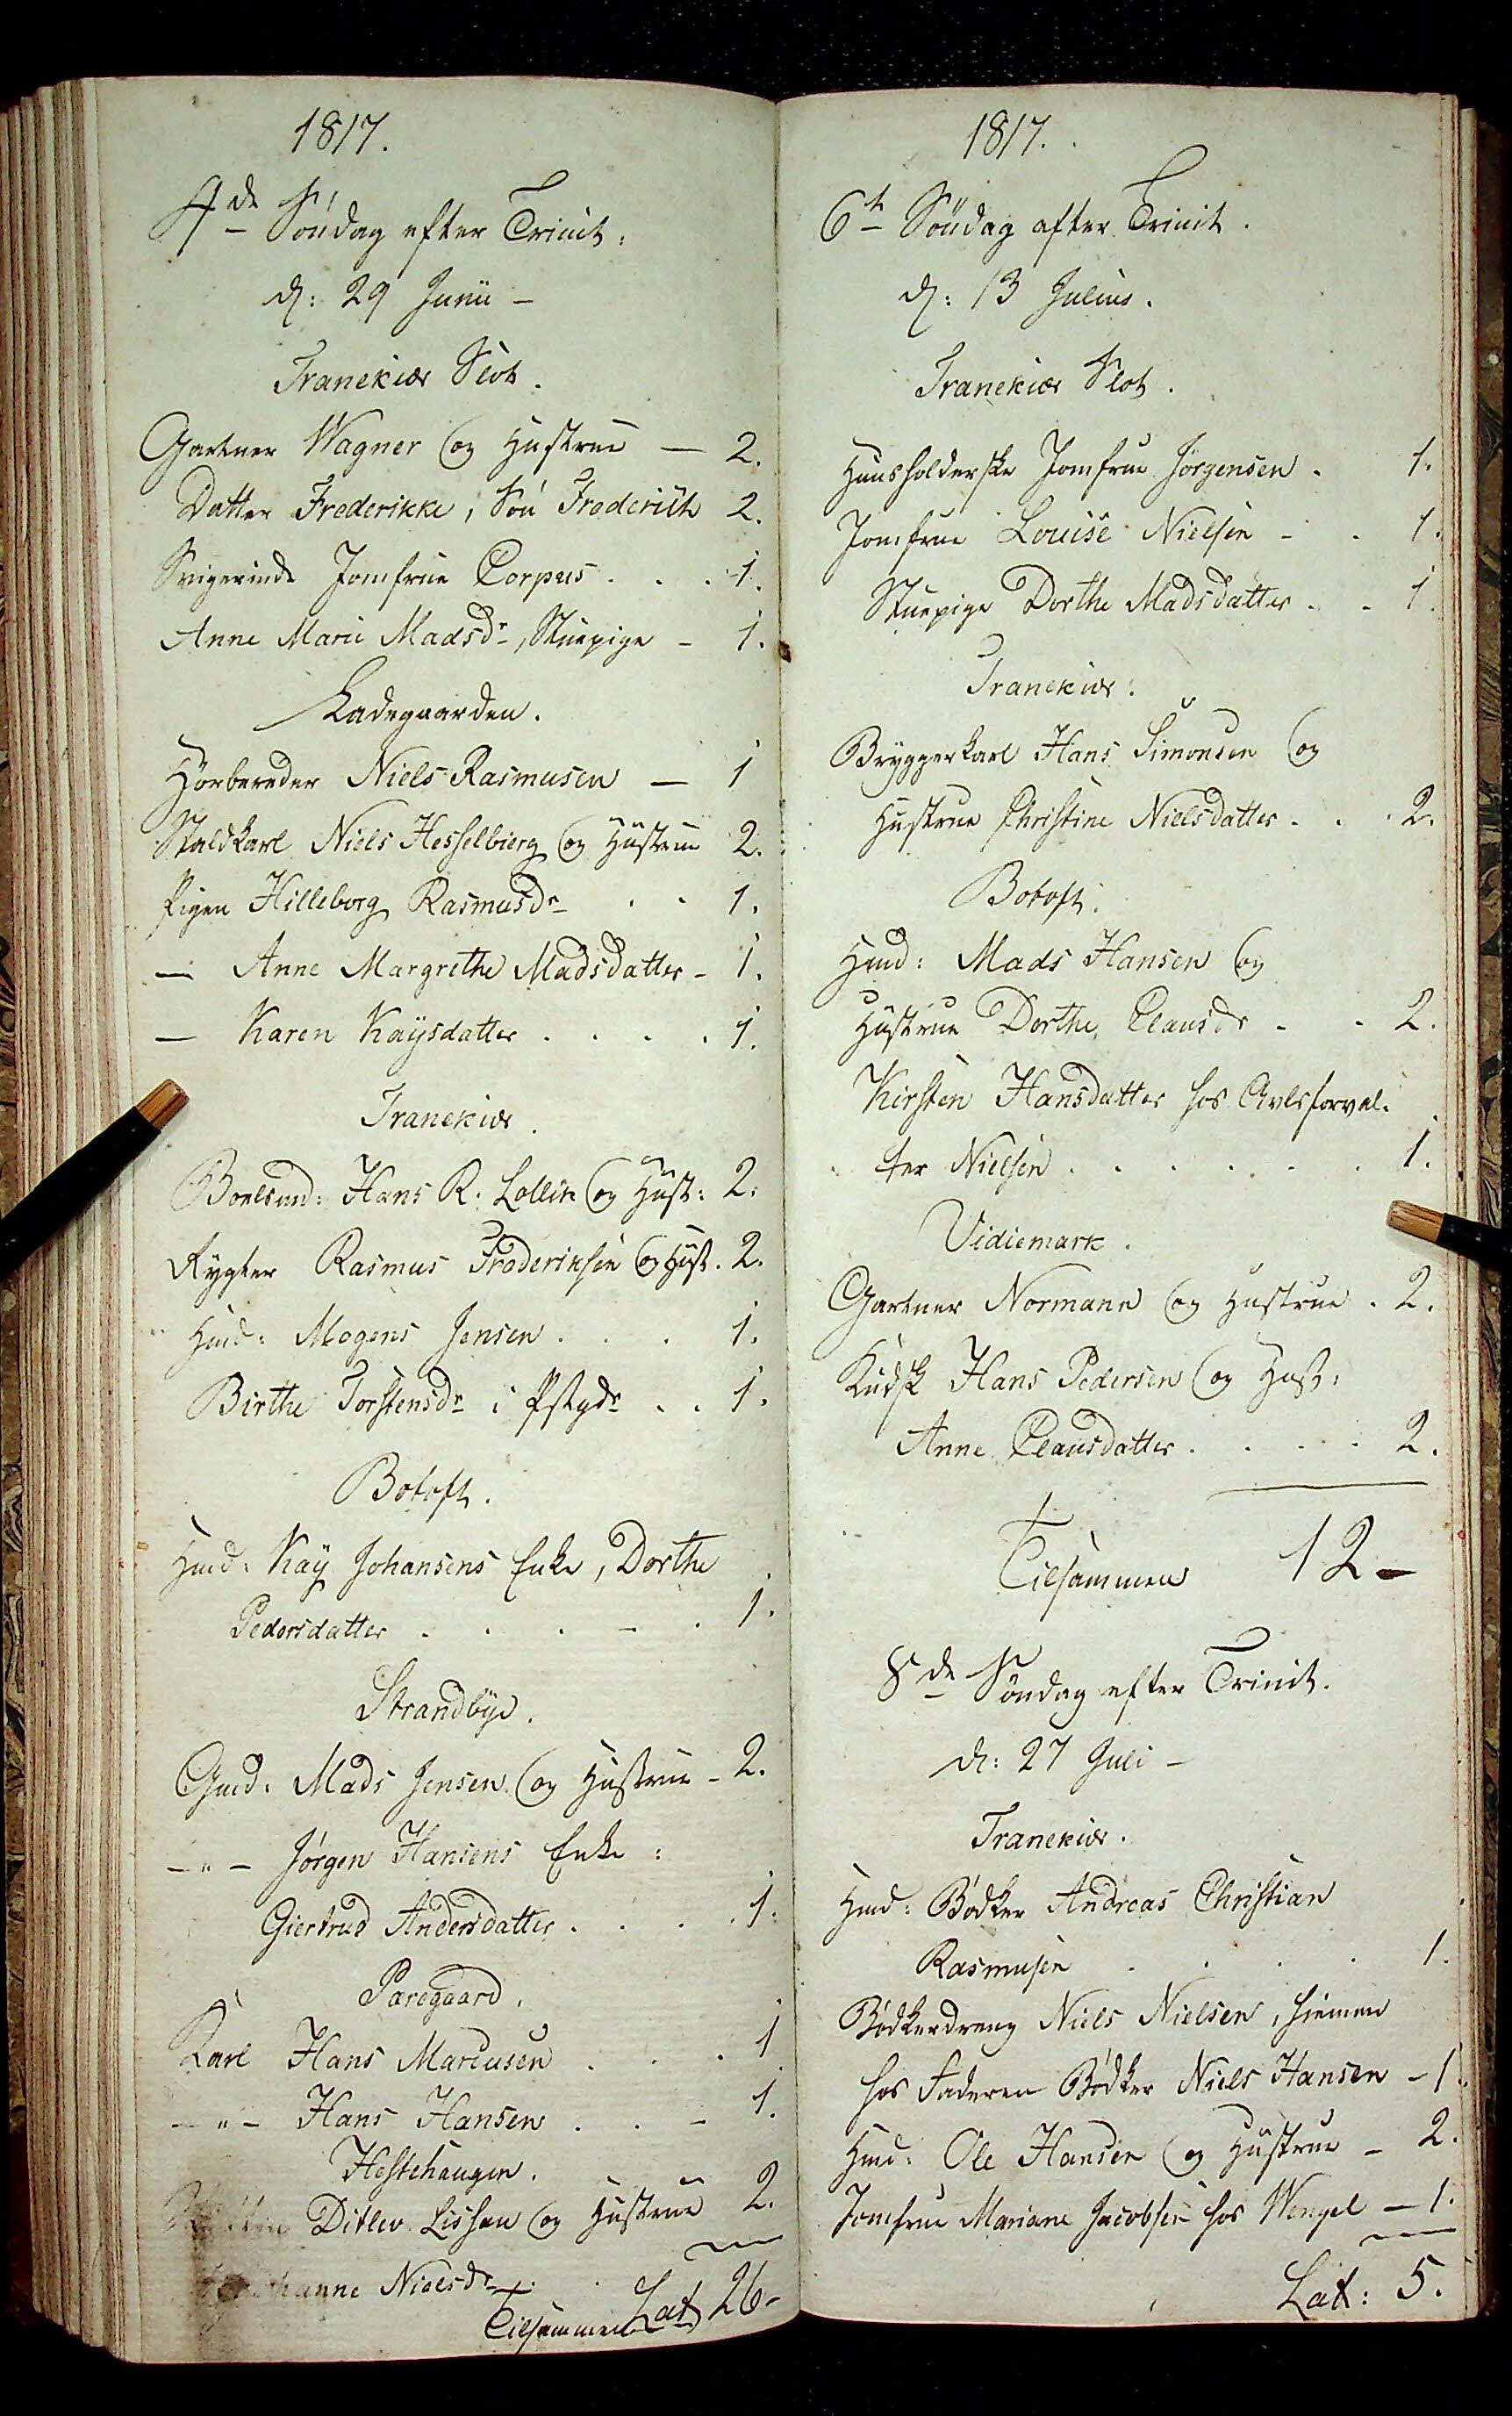1817.
4de Søndag efter Trinit:
d: 29 Junii
Tranekiær Slot.
Gartner Wagner og Hustrue - 2.
Datter Frederikke, Søn Friderich 2.
Svigerinde Jomfrue Corpus . . . 1.
Anne Marie Madsdr Stuepige - 1.
Ladegaarden.
Hørbereder Niels Rasmusen - 1
Staldkarl Niels Hesselbierg og Hustrue 2.
Pigen Hilleborg Rasmusdr . . 1.
- Anne Margrethe Madsdatter - 1.
- Karen Kaysdatter . . . . 1.
Tranekiær.
Boelsmd: Hans R. Lollik og Hust: 2.
Rygter Rasmus Frederiksen og Hust . 2.
Hmd: Mogens Jensen . . . 1
Birthe Torstensdr i Pstgdr . . 1.
Botoft.
Hmd: Kay Johansens Enke, Dorthe
Pedersdatter . . . - . 1
Strandbye.
Gmd: Mads Jensen og Hustrue - 2.
-"- Jørgen Hansens Enke:
Giertrud Andersdatter . . . . 1
Pæregaard.
Karl Hans Marcusen . . . 1
-"- Hans Hansen . . - 1.
Hestehaugen.
Skytten Ditlev Lissau og Hustrue 2.
## Johanne Nielsdr 
Tiksammen Lat 26-
1817.
6te Søndag efter Trinit.
d: 13 Juli##.
Tranekiær Slot.
Huusholderske Jomfrue Jørgensen . 1.
Jomfrue Louise Nielsen . . 1.
Stuepige Dorthe Madsdatter . . 1.
Tranekiær.
Bryggerkarl Hans Simonsen og
Hustrue Christine Nielsdatter . . 2.
Botoft.
Hmd: Mads Hansen og
Hustrue Dorthe Clausdr . . 2.
Kirsten Hansdatter hos Avlsforval¬
ter Nielsen . . . . . . . 1.
Vidiemark.
Gartner Normann og Hustrue . 2.
Kudsk Hans Pedersen og Hust:
Anne Clausdatter . . . . 2.
Tilisammen 12-
8de Søndag efter Trinit.
d: 27 Juli
Tranekiær.
Hmd: Bødker Andreas Christian
Rasmusen . . . . 1.
Bødkerdreng Niels Nielsen, hiemme
hos Faderen Bødker Niels Hansen -1
Hmd: Ole Hansen og Hustrue - 2
Jomfrue Mariane Jacobsen hos Wengel - 1
Lat: 5.
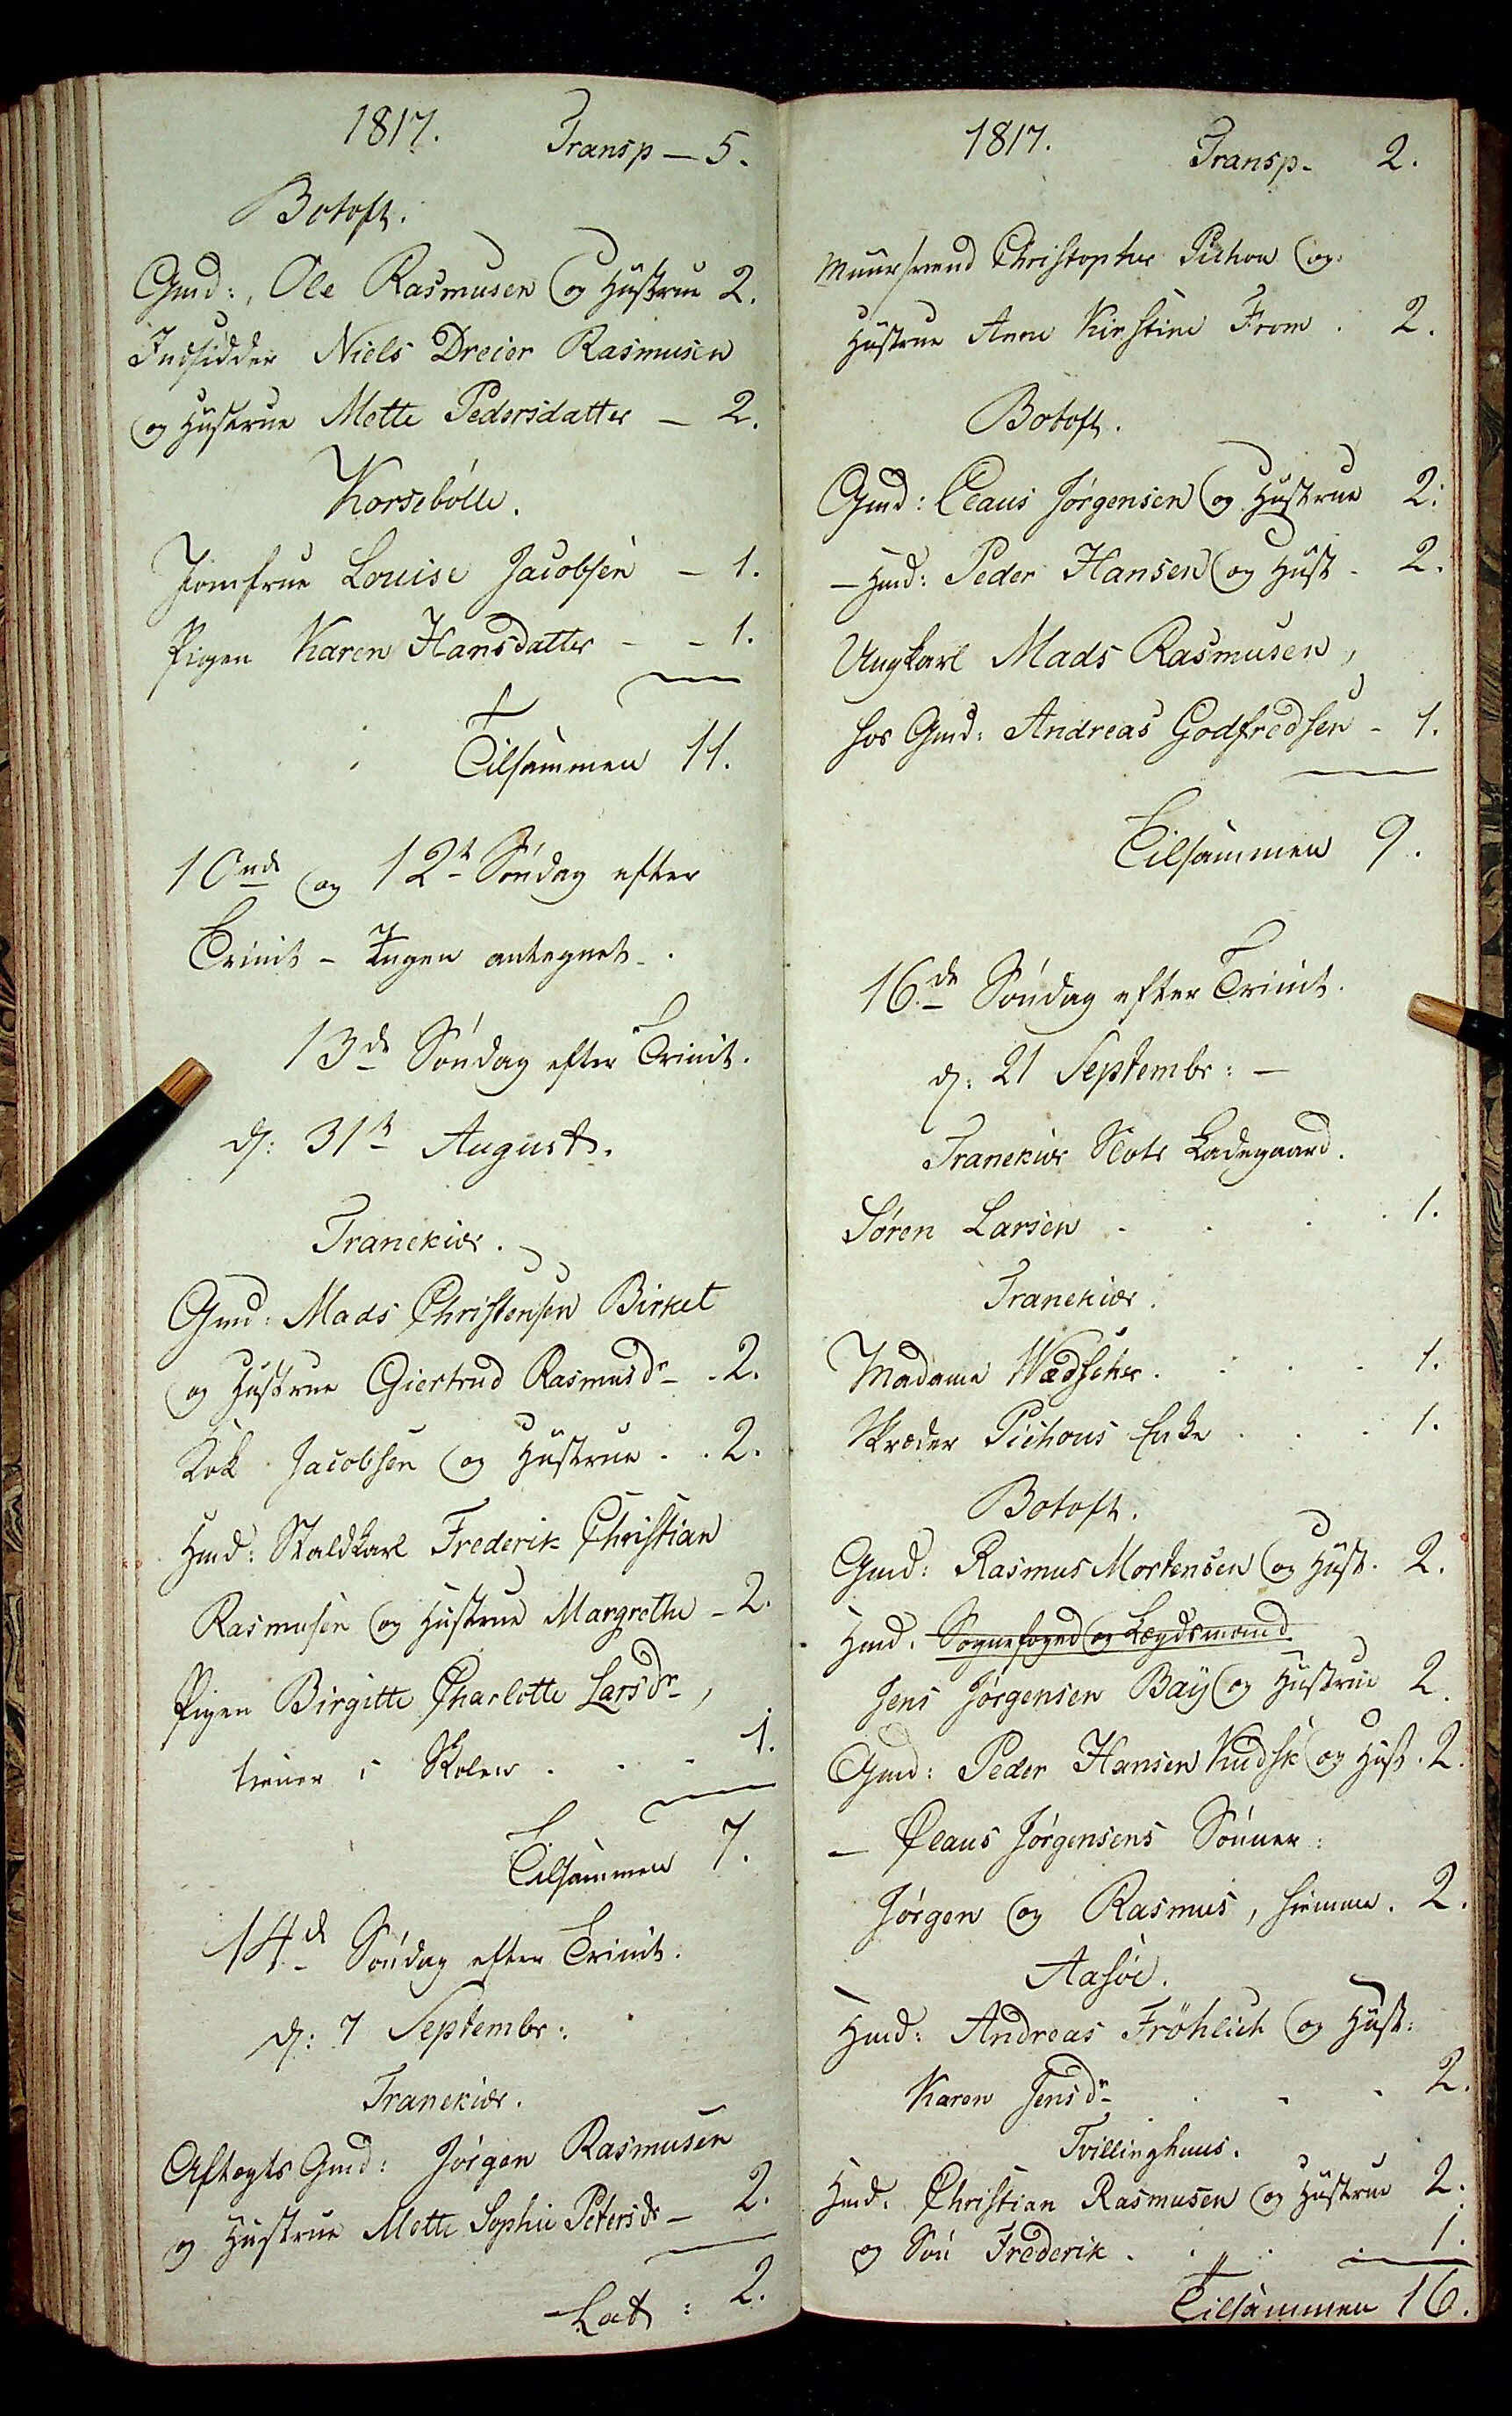1817.
Transp - 5.
Botoft.
Gmd: Ole Rasmusen og Hustrue 2.
Indsidder Niels Dreier Rasmusen
og Hustrue Mette Pedersdatter - 2.
Korsebølle.
Jomfrue Louise Jacobsen - 1.
Pigen Karen Hansdatter - - 1.
Tilsammen 11.
10nde og 12te Søndag efter
Trinit - Inger antaget.
13de Søndag efter Trinit.
d: 31te August.
Tranekiær.
Gmd: Mads Christensen Birket
og Hustrue Giertrud Rasmusdr . 2.
Kok Jacobsen og Hustrue . . 2.
Hmd: Staldkarl Frederik Christian
Rasmusen og Hustrue Margrethe - 2.
Pigen Birgitte Charlotte Larsdr
tiener i Skolen . . . 1.
Tilsammen 7
14de Søndag efter Trinit.
d: 7 Septembr:
Tranekiær.
Aftægts Gmd: Jørgen Rasmusen
og Hustrue Mette Sophia Pedersdr - 2
Lat: 2.
1817.
Transp - 2.
Muursvend Christopher Pichou og
Hustrue Anne Kirstind From . 2.
Botoft.
Gmd: Claus Jørgensen og Hustrue 2:
Hmd: Peder Hansen og Hust . 2.
Ungkarl Mads Rasmusen,
hos Gmd: Andreas Godfredsen - 1.
Tilsammen 9.
16de Søndag efter Trinit.
d: 21 Septembr:
Tranekiær Slots Ladegaard.
Søren Larsen . . . . 1.
Tranekiær.
Madame Wadscher . . . . 1.
Skræder Pichous Enke . . . 1.
Botoft.
Gmd: Rasmus Mortensens og Hust . 2.
Hmd: Sognefoged og Lægdsmand
Jens Jørgensen Bay og Hustrue 2.
Gmd: Peder Hansen Kudsk og Hust . 2.
- Claus Jørgensens Sønner:
Jørgen og Rasmus, hiemme . 2.
Aasøe.
Hmd: Andreas Frøhlich og Hust:
Karen Jensdr . . .  2.
Tvillinghuus.
Hmd. Christian Rasmusen og Hustrue 2.
og Søn Friderik . . . . 1.
Tilsammen 16.